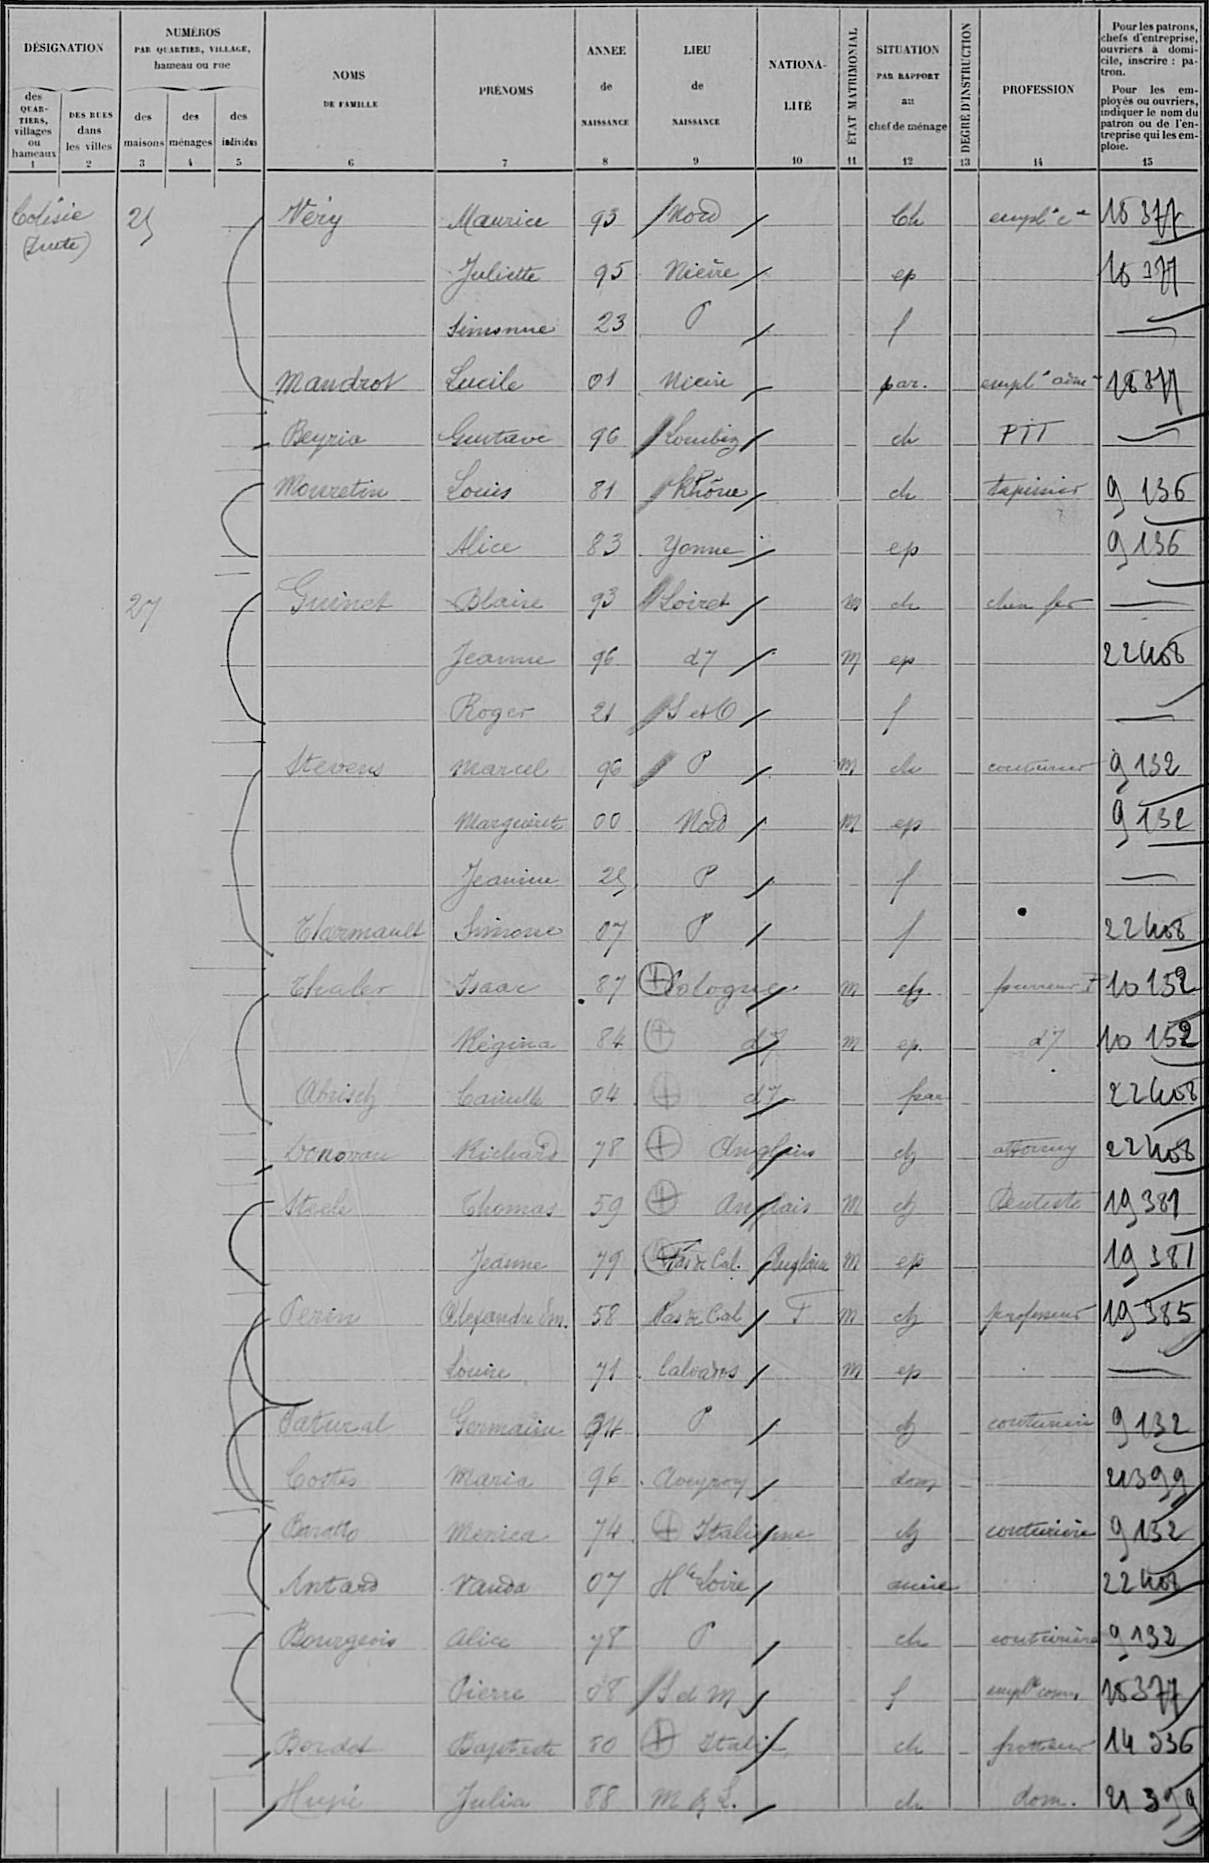Véry/Maurice/93/Nord/¤/¤/Ch/¤/empl C/18377
¤/Juliette/95/Nièvre/¤/¤/ep/¤/¤/18377
¤/Simonne/23/P/¤/¤/f/¤/¤/¤
Maudrot/Lucile/01/Nièvre/¤/¤/par /¤/empl admi/18377
Beyrica/Gustave/96/Lourbig/¤/¤/ch/¤/PTT/¤
Mouretin/Louis/81/Rhône/¤/¤/ch/¤/tapissier/9136
¤/Alice/83/Yonne/¤/¤/ep/¤/¤/9136
Guinet/Blaise/93/Loiret/¤/m/ch/¤/chem fer/¤
¤/Jeanne/96/d°/¤/m/ep/¤/¤/22408
¤/Roger/21/S et O/¤/¤/f/¤/¤/¤
Stevens/Marcel/96/P/¤/m/ch/¤/couturier/9132
¤/Marguerite/00/Nord/¤/M/ep/¤/¤/9132
¤/Jeanine/28/P/¤/¤/f/¤/¤/¤
Clarmault/Simone/07/P/¤/¤/f/¤/¤/22408
Thaler/Isaac/87/Pologne/¤/M/ep/¤/fourreur P/10152
¤/Régina/84/d°/¤/m/ep /¤/d°/10152
Abrisch/Camille/04/d°/¤/¤/par/¤/¤/22408
Donovan/Richard/78/Anglais/¤/¤/ch/¤/attorney/22408
Steele/Thomas/59/Anglais/¤/M/ch/¤/Dentiste/19381
¤/Jeanne/79/Pas de Cal/Anglaise/M/ep/¤/¤/19381
Perin/Alexandre Em /58/Pas de Cal/F/m/ch/¤/professeur/19385
¤/Louise/71/Calvados/¤/m/ep/¤/¤/¤
Paturat/Germain/94/P/¤/¤/ch/¤/couturière/9132
Costes/Maria/96/Aveyron/¤/¤/dom/¤/¤/21399
Paratto/Monica/74/Italienne/¤/¤/ch/¤/couturière/9132
Antard/Vauda/07/Hte Loire/¤/¤/amie/¤/¤/22408
Bourgeois/Alice/78/P/¤/¤/ch/¤/couturière/9132
¤/Pierre/08/S et M/¤/¤/f/¤/empl com/18377
Bordet/Baptiste/80/Italie/¤/¤/ch/¤/frotteur/14936
Hupré/Julia/88/M & L /¤/¤/ch/¤/dom /21399
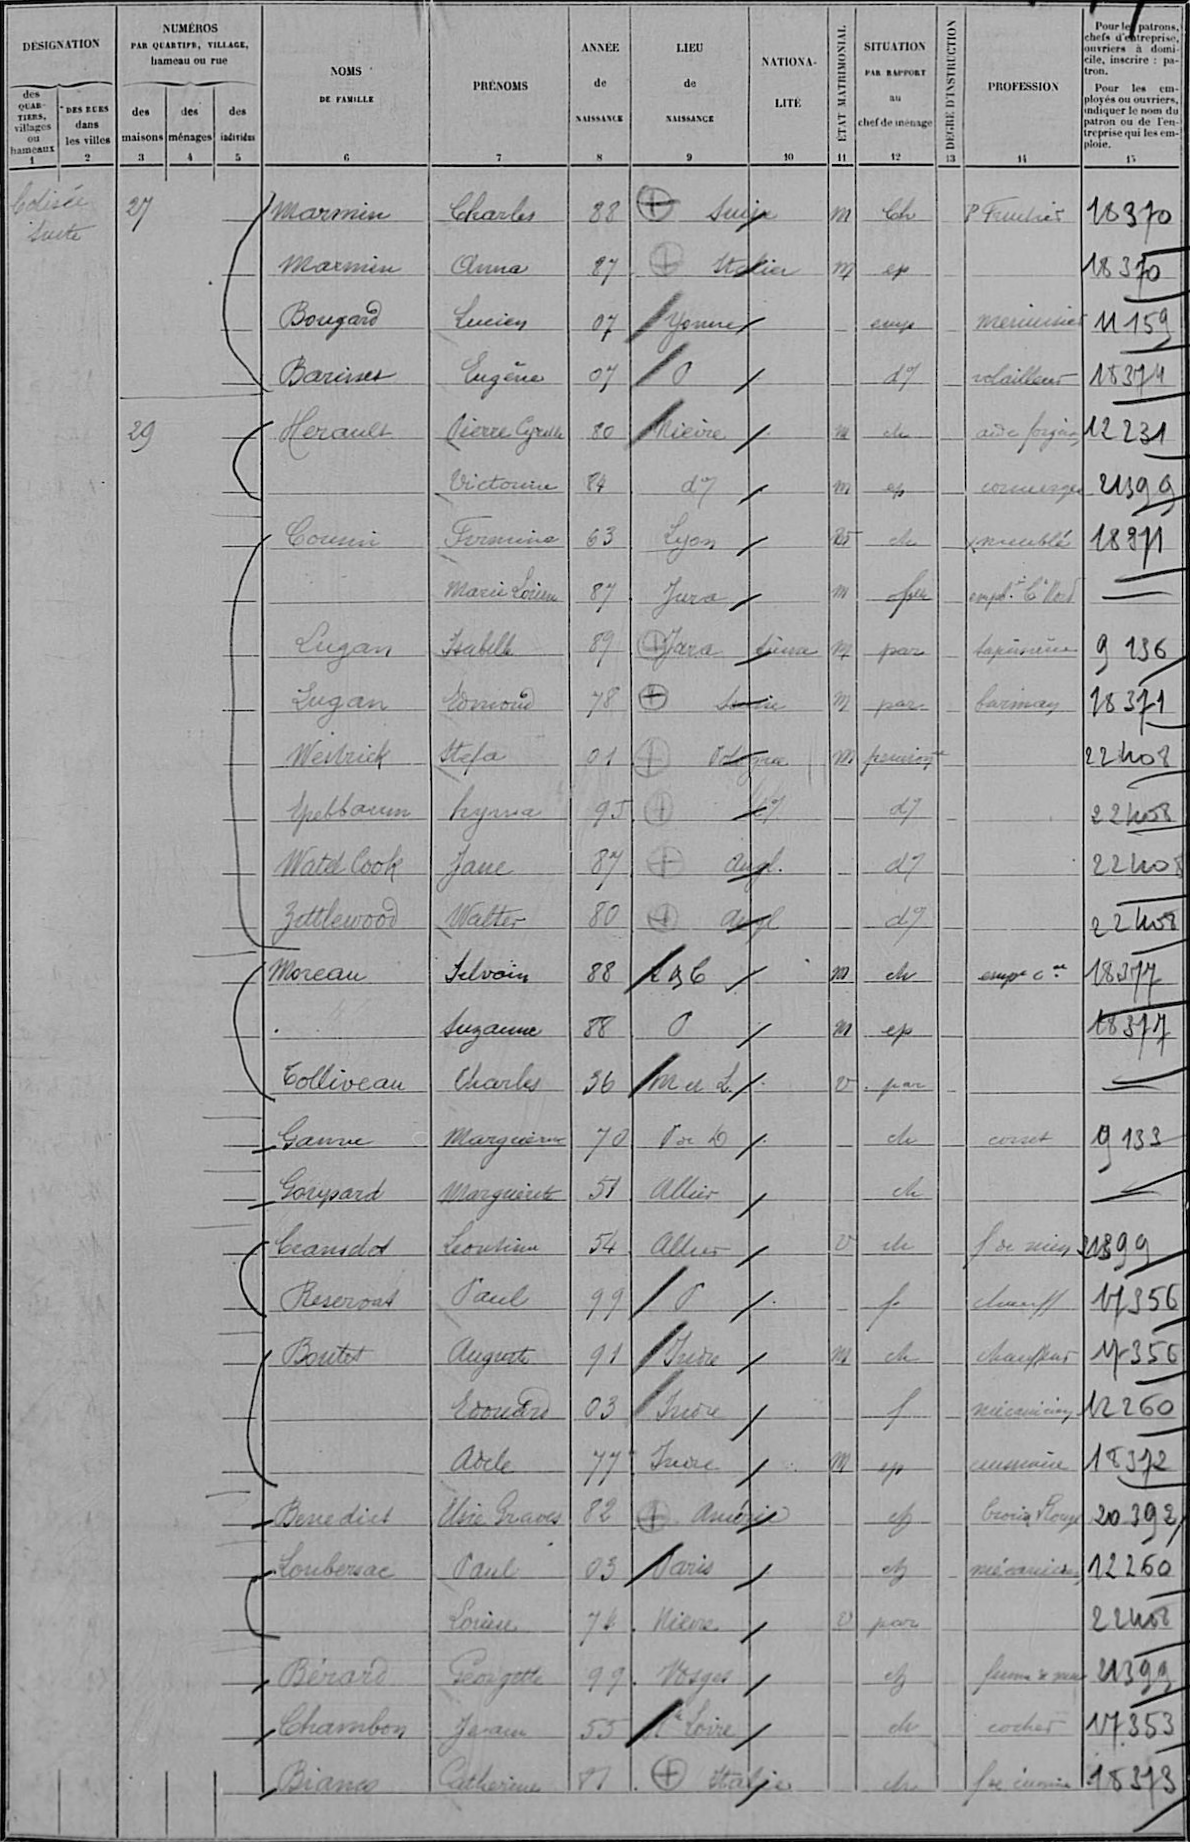Marmin/Charles/88/Suisse/¤/M/Ch/¤/P Fruitier/18370
Marmin/Anna/87/Italien/¤/M/ep/¤/¤/18370
Bougard/Lucien/07/Yonne/¤/¤/emp/¤/menuisier/11159
Barisset/Eugène/07/P/¤/¤/d°/¤/volailleur/18374
Herault/Pierre Cyrille/80/Nièvre/¤/M/ch/¤/aide forgeron/12231
¤/Victorine/84/d°/¤/M/ep/¤/concierge/21399
Cousin/Francine/63/Lyon/¤/V/ch/¤/meublé/18371
¤/Marie Louise/87/Jura/¤/M/fille/¤/emplé C Nord/¤
Lugan/Isabelle/89/Jura/Suisse/m/par/¤/tapissière/9136
Lugan/Edmond/78/Suisse/¤/m/par/¤/barman/18371
Weitrick/Stefa/01/Pologne/¤/m/pensionre/¤/¤/22408
Epebbaum/hyrria/95/d°/¤/¤/d°/¤/¤/22408
Watel Cook/Jane/87/Angl/¤/¤/d°/¤/¤/22408
Zettlewood/Walter/80/Angl/¤/¤/d°/¤/¤/2208
Moreau/Silvain/88/L & C/¤/m/ch/¤/empé Cce/18377
¤/Suzanne/88/P/¤/M/ep/¤/¤/18377
Tolliveau/Charles/36/M et L /¤/v/par/¤/¤/¤
Gaurie/Marguerite/70/P de D/¤/¤/ch/¤/corset/9133
Gorypard/Marguerite/51/Allier/¤/¤/ch/¤/¤/¤
Transdet/Leontine/54/Allier/¤/V/ch/¤/f de men/21399
Reservas/Paul/99/P/¤/¤/f /¤/chauff/17356
Boutet/Auguste/91/Indre/¤/M/ch/¤/chauffeur/17356
¤/Edouard/03/Indre/¤/¤/f/¤/mécanicien/12260
¤/Adele/77/Indre/¤/M/ep/¤/cuisinière/18372
Benedict/Elsie Graves/82/Améric/¤/¤/ep/¤/Croix rouge/20392
Loubersac/Paul/03/Paris/¤/¤/ch/¤/mécanicien/12260
¤/Louise/71/Nièvre/¤/V/par/¤/¤/22408
Bérard/Georgette/99/Vosges/¤/¤/ch/¤/femme de men/21399
Chambon/Jean/55/Hte Loire/¤/¤/ch/¤/cocher/17353
Bianco/Catherine/87/Italie/¤/¤/ch/¤/f de cuisine/18373